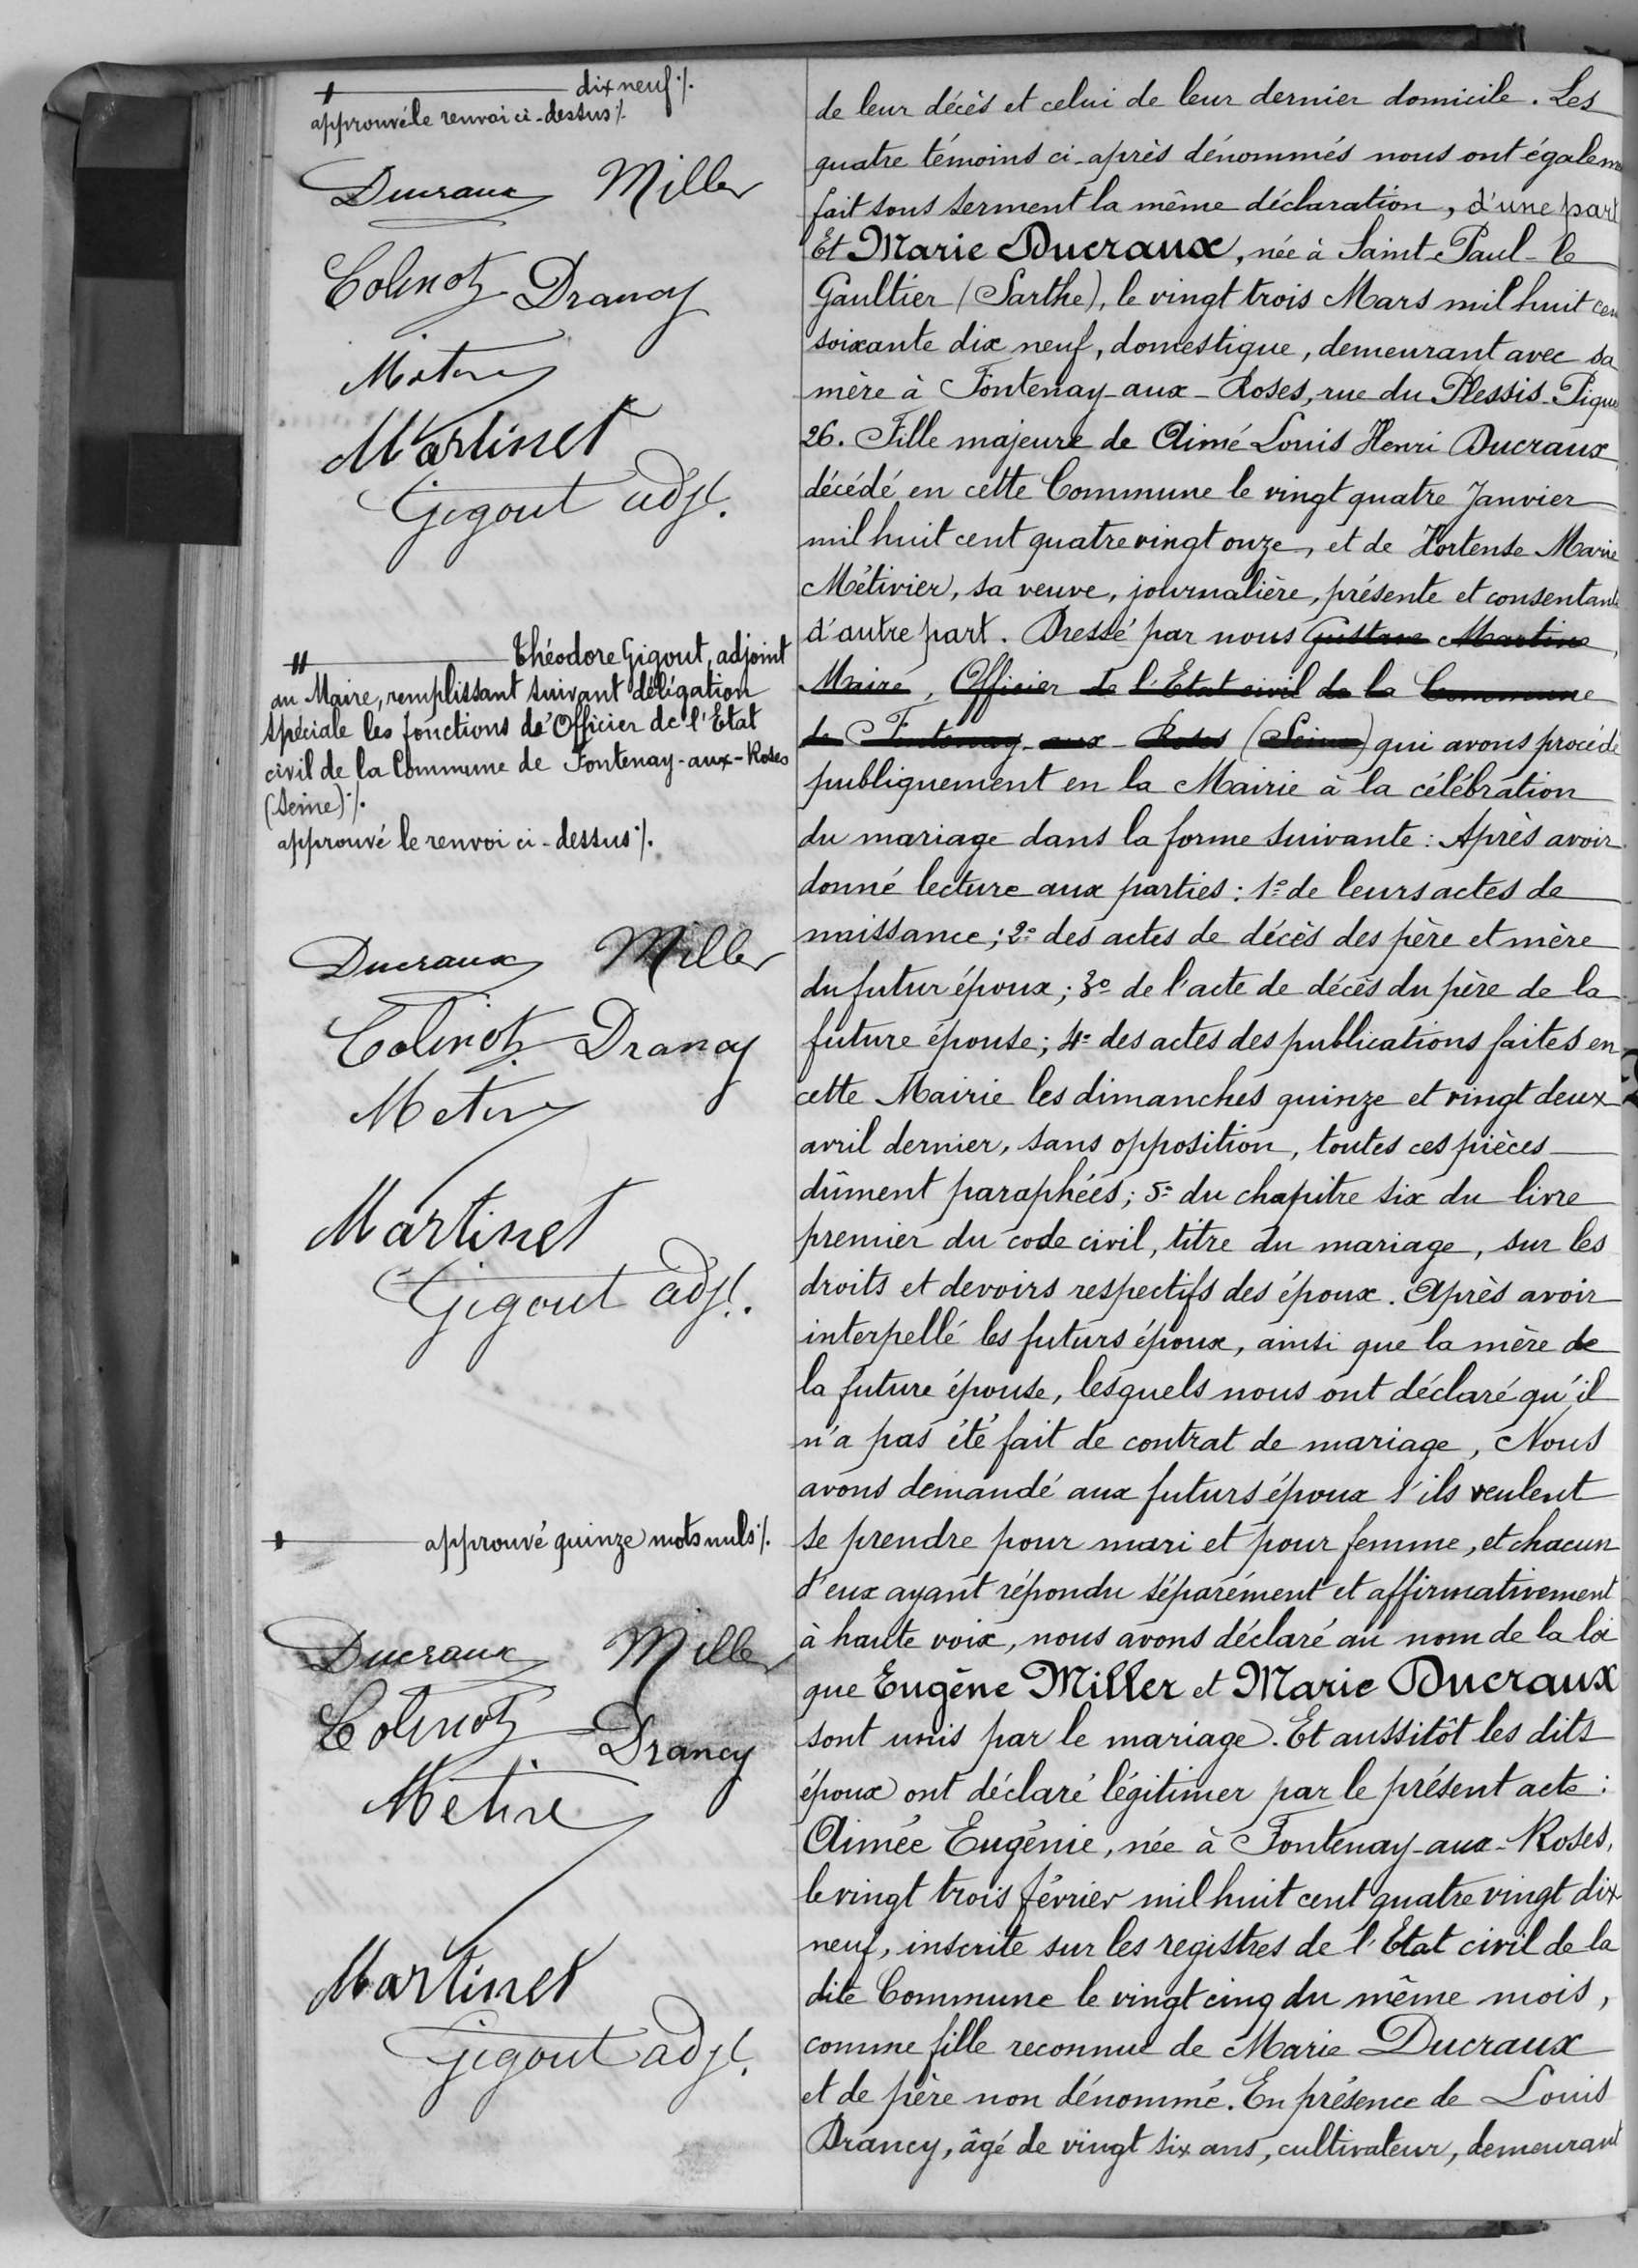de leur décès et celui de leur dernier domicile. Les
quatre témoins ci-après dénommés nous ont égalem
fait sous serment la même déclaration, d'une part
Et Marie Ducraux, née à Saint Paul-le
Gaultier (Sarthe), le vingt trois Mars mil huit cent
soixante dix neuf, domestique, demeurant avec sa
mère à Fontenay-aux-Roses, rue du Plessis Pique
26. Fille majeure de Aimé Louis Henri Ducraux
décédé en cette Commune le vingt quatre Janvier
mil huit cent quatre vingt onze, et de Hortense Marie
Métivier, sa veuve, journalière, présente et consentante
d'autre part. Dressé par nous, Gustave Martin
Maire, Officier de l'Etat civil de la Commune
de Fontenay-aux-Roses (Seine) qui avons procédé
publiquement en la Mairie à la célébration
du mariage dans la forme suivant: Après avoir
donné lecture aux parties: 1° de leurs actes de
naissance; 2° des actes de décès du père de la
future épouse; 4° des actes des publications faites en
cette Mairie les dimanches quinze et vingt deux
avril dernier, sans opposition, toutes ces pièces
dûment paraphées; 5° du chapitre six du livre
premier du code civil, titre du mariage, sur les
droits et devoirs respectifs des époux. Après avoir
interpellé les futurs époux, ainsi que la mère de
la future épouse, lesquels nous ont déclaré qu'il
n'a pas été fait de contrat de mariage, Nous
avons demandé aux futurs époux, s'ils veulent
se prendre pour mari et pour femme, et chacun
d'eux ayant répondu séparément et affirmativement
à haute voix, nous avons déclaré au nom de la loi
que Eugène Miller et Marie Ducraux
sont unis par le mariage. Et aussitôt les dits
époux ont déclaré légitimer par le présent acte:
Aimée Eugénie, née à Fontenay-aux-Roses,
le vingt trois février mil huit cent quatre vingt dix
neuf, inscrite sur les registres de l'Etat civil de la
dite Commune le vingt cinq du même mois,
comme fille reconnue de Marie Ducraux
et de père non dénommé. En présence de Louis
Drancy, âgé de vingt six ans, cultivateur, demeurant
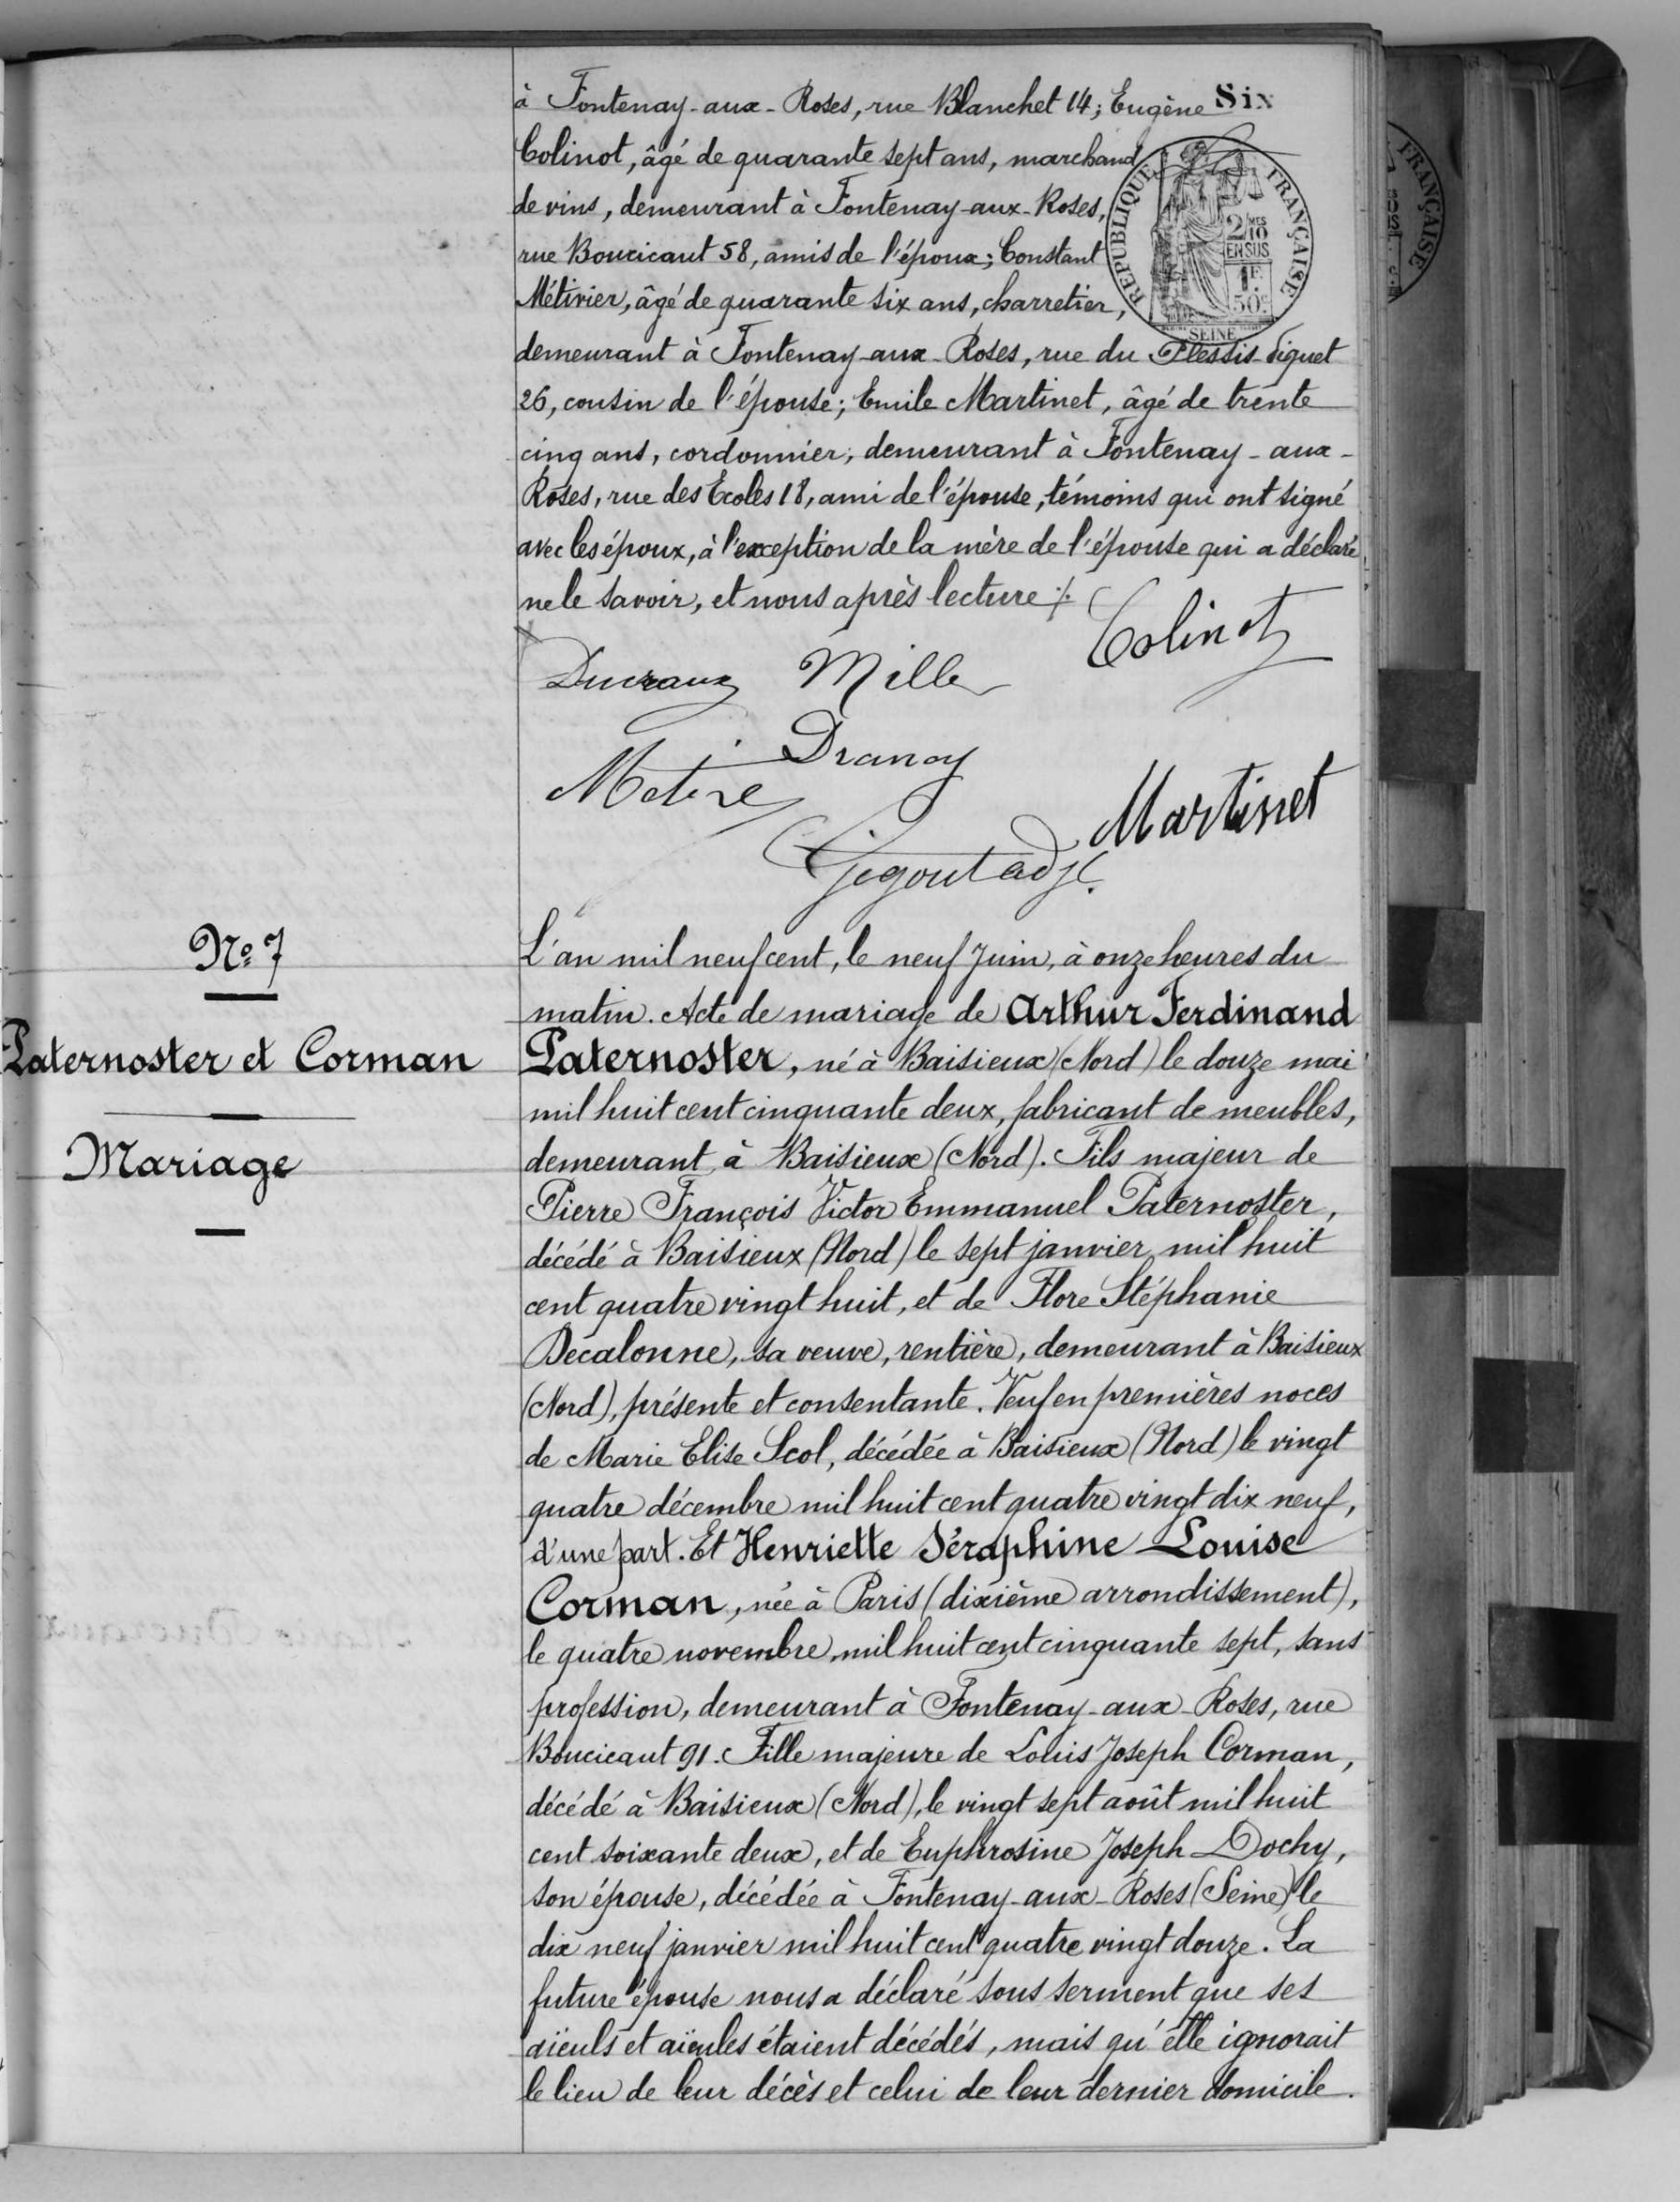à Fontenay-aux-Roses, rue Blanchet 14; Eugène
Colinot, âgé de quarante sept ans, marchand
de vins, demeurant à Fontenay-aux-Roses,
rue Boucicaut 58, amis de l'époux; Constant
Métinier, âgé de quarante six ans, charretier,
demeurant à Fontenay-aux-Roses, rue du Plessis-Viquet
26, cousin de l'épouse; Emile Martiner, âgé de trente
cinq ans, cordonnier; demeurant à Fontenay-aux-
Roses, rue des Ecoles 18, ami de l'épouse, témoins qui ont signé
avec les époux, à l'exception de la mère de l'épouse qui a déclaré
ne le savoir, et nous après lecture./.
N°7
Paternoster et Corman
Mariage
L'an mil neuf cent, le neuf Juin, à onze heures du
matin. Acte de mariage de Arthur Ferdinand
Paternoster, né à Baisieux (Nord) le douze mai
mil huit cent cinquante deux, fabricant de meubles,
demeurant à Baisieux (Nord). Fils majeur de
Pierre François Victor Emmanuel Paternoster,
décédé à Baisieux (Nord) le sept janvier mil huit
cent quatre vingt huit, et de Flore Séphanie
Decalonne, sa veuve, rentière, demeurant à Baisieux
(Nord), présente et consentante. Veuf en premières noces
de Mari Elise Scol, décédée à Baisieux (Nord) le vingt
quatre décembre mil huit cent quatre vingt dix neuf,
d'une part. Et Henriette Séraphine Louis
Corman, née à Paris (dixième) arrondissement),
le quatre novembre mil huit cent cinquante sept, sans
profession, demeurant à Fontenay-aux-Roses, rue
Boucicaut 91. Fille majeure de Louis Joseph Corman,
décédé à Baisieux (Nord), le vingt sept août mil huit
cent soixante deux, et de Euphrosine Joseph Dochy,
son épouse, décédée à Fontenay-aux-Roses (Seine) le
dix neuf janvier mil huit cent quatre vingt douze. La
future épouse nous a déclaré sous serment que ses
aïeuls et aïeules étaient décédés, mais qu'elle ignorait
le lieu de leur décès et celui de leur dernier domicile.